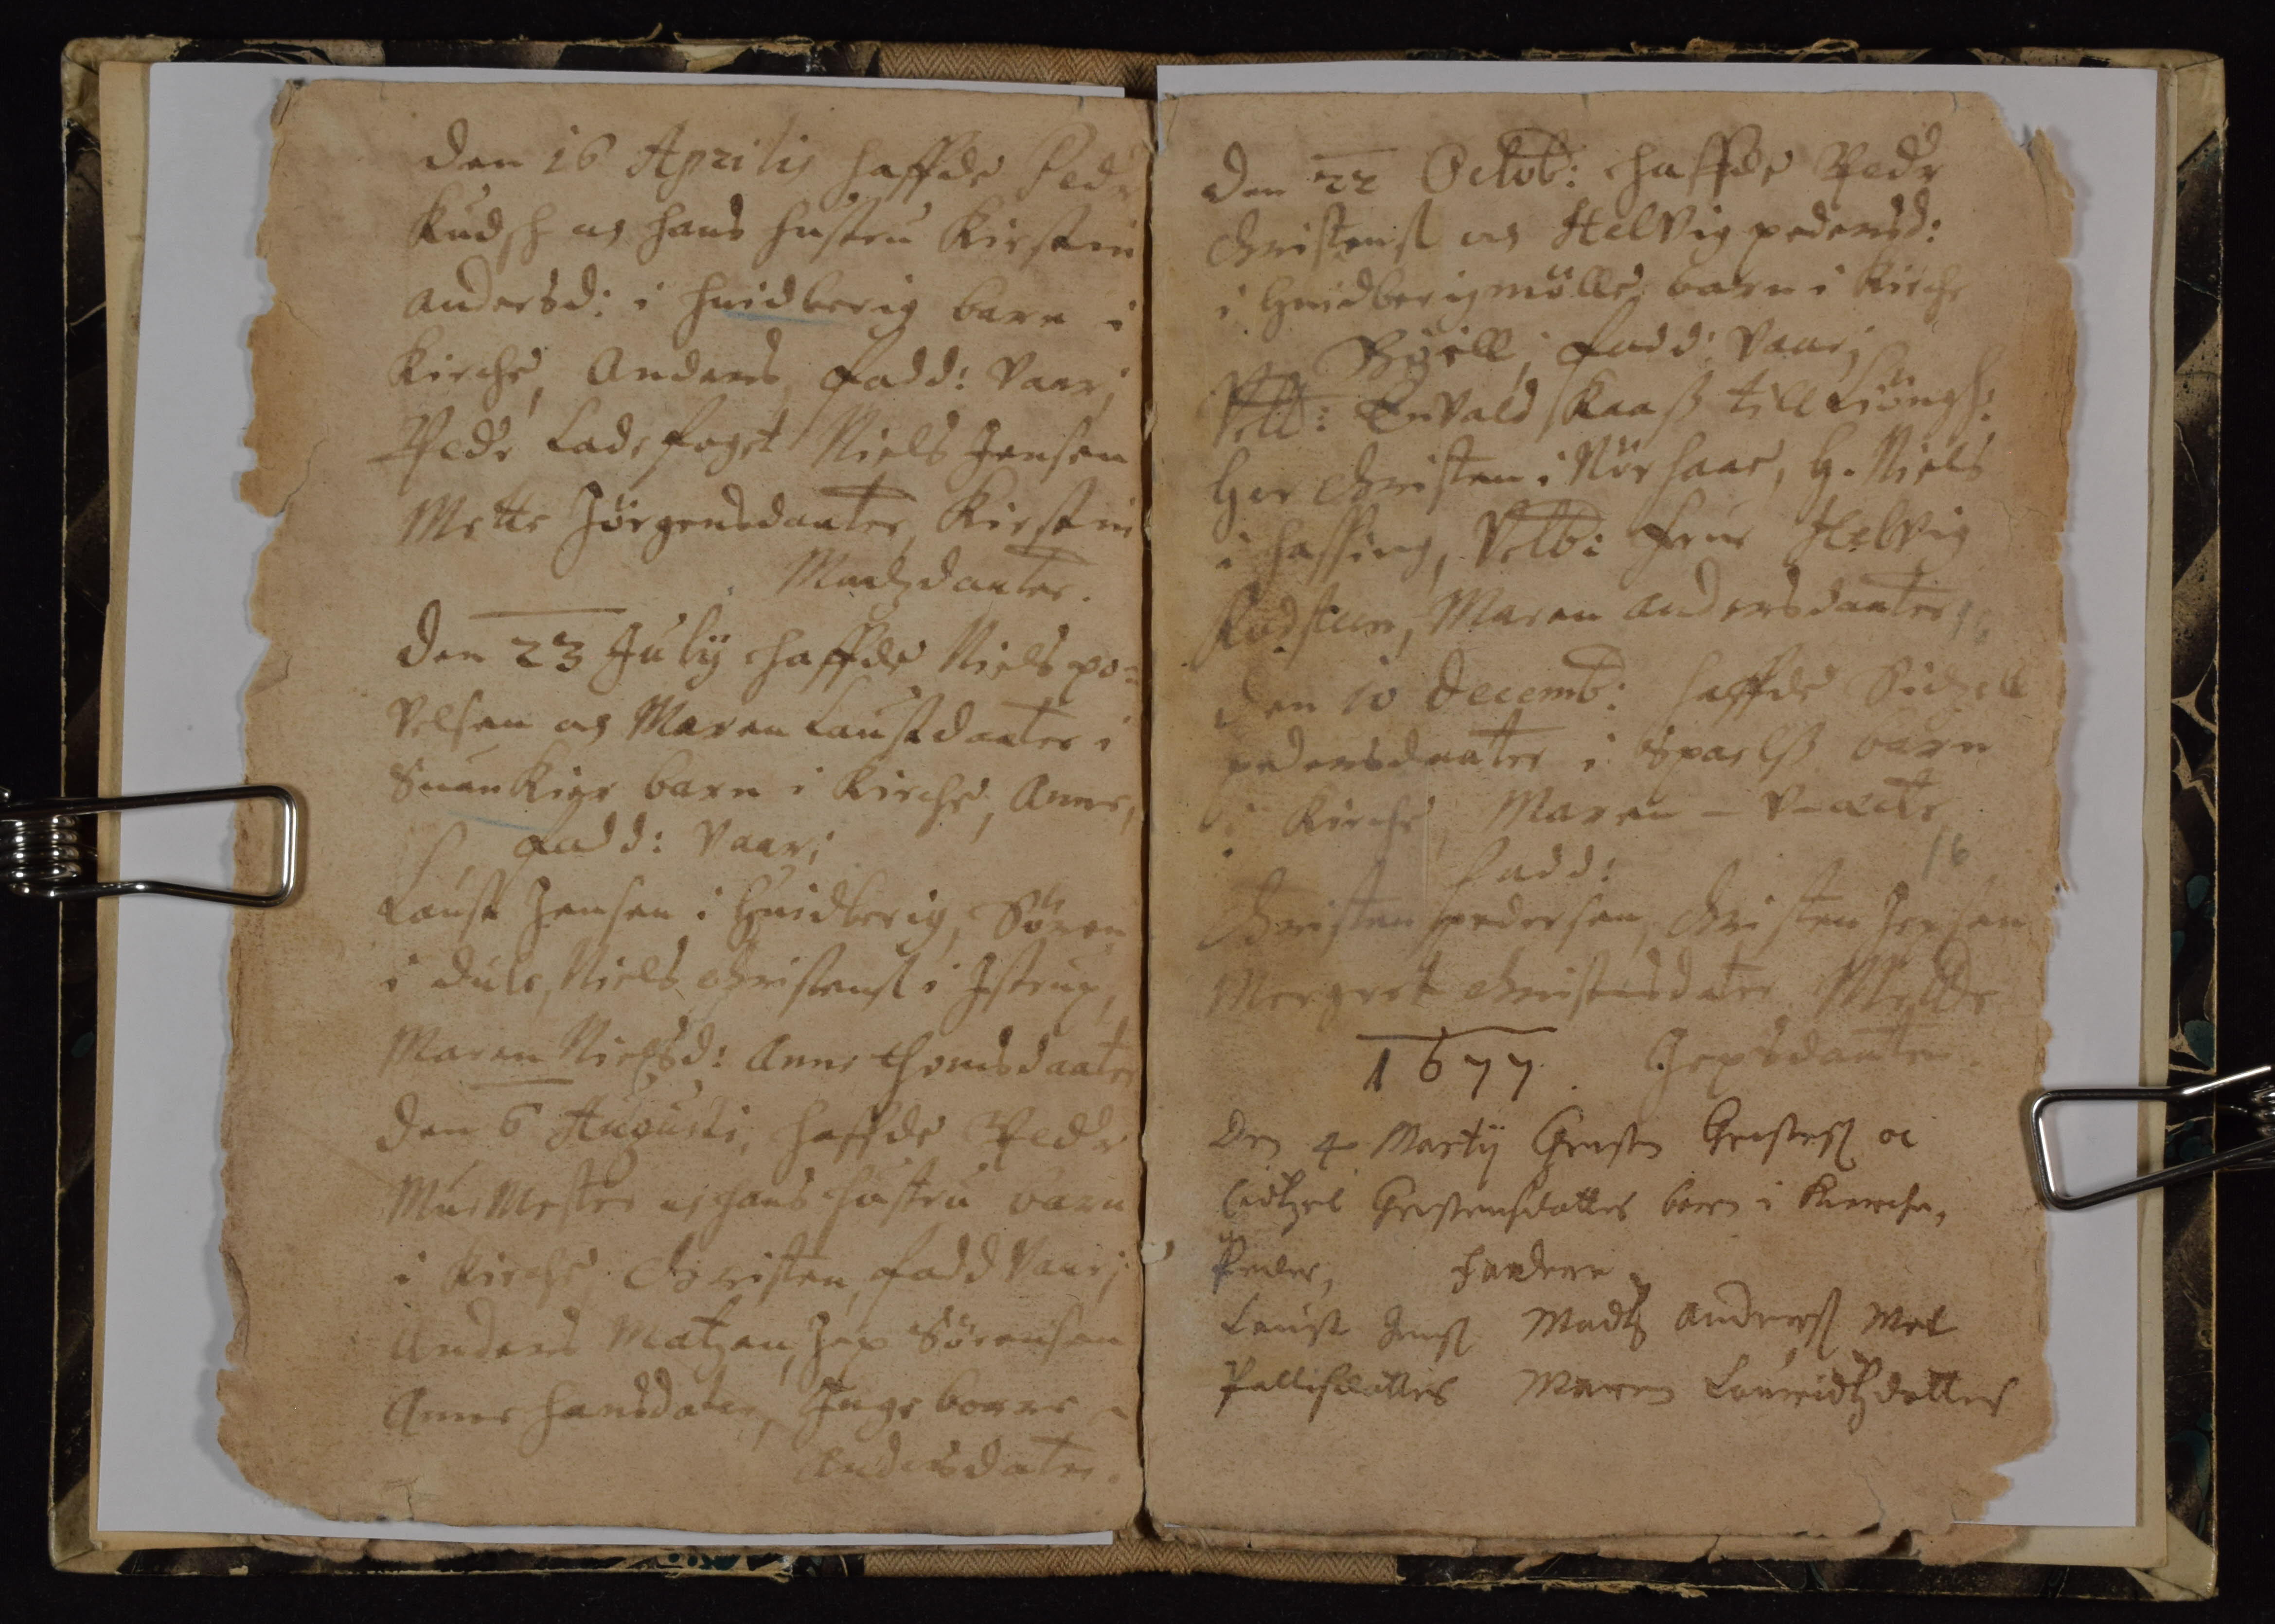Den 16 Aprilis haffde Pedr
kudsh och hans hustru Kirstin
Andersd: i hvidberig barn i
Kirche, Anders, Fadd: vaar
Pedr Ladefoget Niels Jensen
Mette Jørgensdauter Kirstin
Madzdauter.
Den 23 Julij haffde Niels po¬
velsen och Maren Laustdaater i
Suankier barn i Kirche, Anne,
Fadd: vaar;
Laust Jensen i Hvidberig, Søren
i Dule Niels christens i Istrup.
Maren Nielsd: Anne Thomsdaater
Den 6 Augusti, haffde Pedr
Mur Mester och hans hustru barn
i Kirche christen, Fadd vaar;
Anderis Matzen Jep Sørensen
Anne Hansdater Ingeborre
Andersdater
Den 22 Octob: Haffde Pedr
Christens och Helvig Pedersd:
i Hvidberig mølle barn i Kirche
Boell Fadd: vaar
Velb: Envold Kaass till Liøngh:
Her Christen i Nørhaae, H. Niels
i Hassing, Velb: Frue Helvig
Radsteen, Maren Andersdaater
Den 10 Decemb: haffde Sidzell
Pedersdaater i Spaelss barn
i Kirche, Maren - v-eccte
Fadd:
christen Pedersen, christens Jepsen
Mergret christesdater Mette
Jepsdauter
1677
Den 4 Martij Cersten Christensn oc
Cidtzel Christensdatter barn i Kierche,
Peder Faddere
Laust Jens Madtz Anders, Met
Pallisdatter Maren Lauridtzdatter
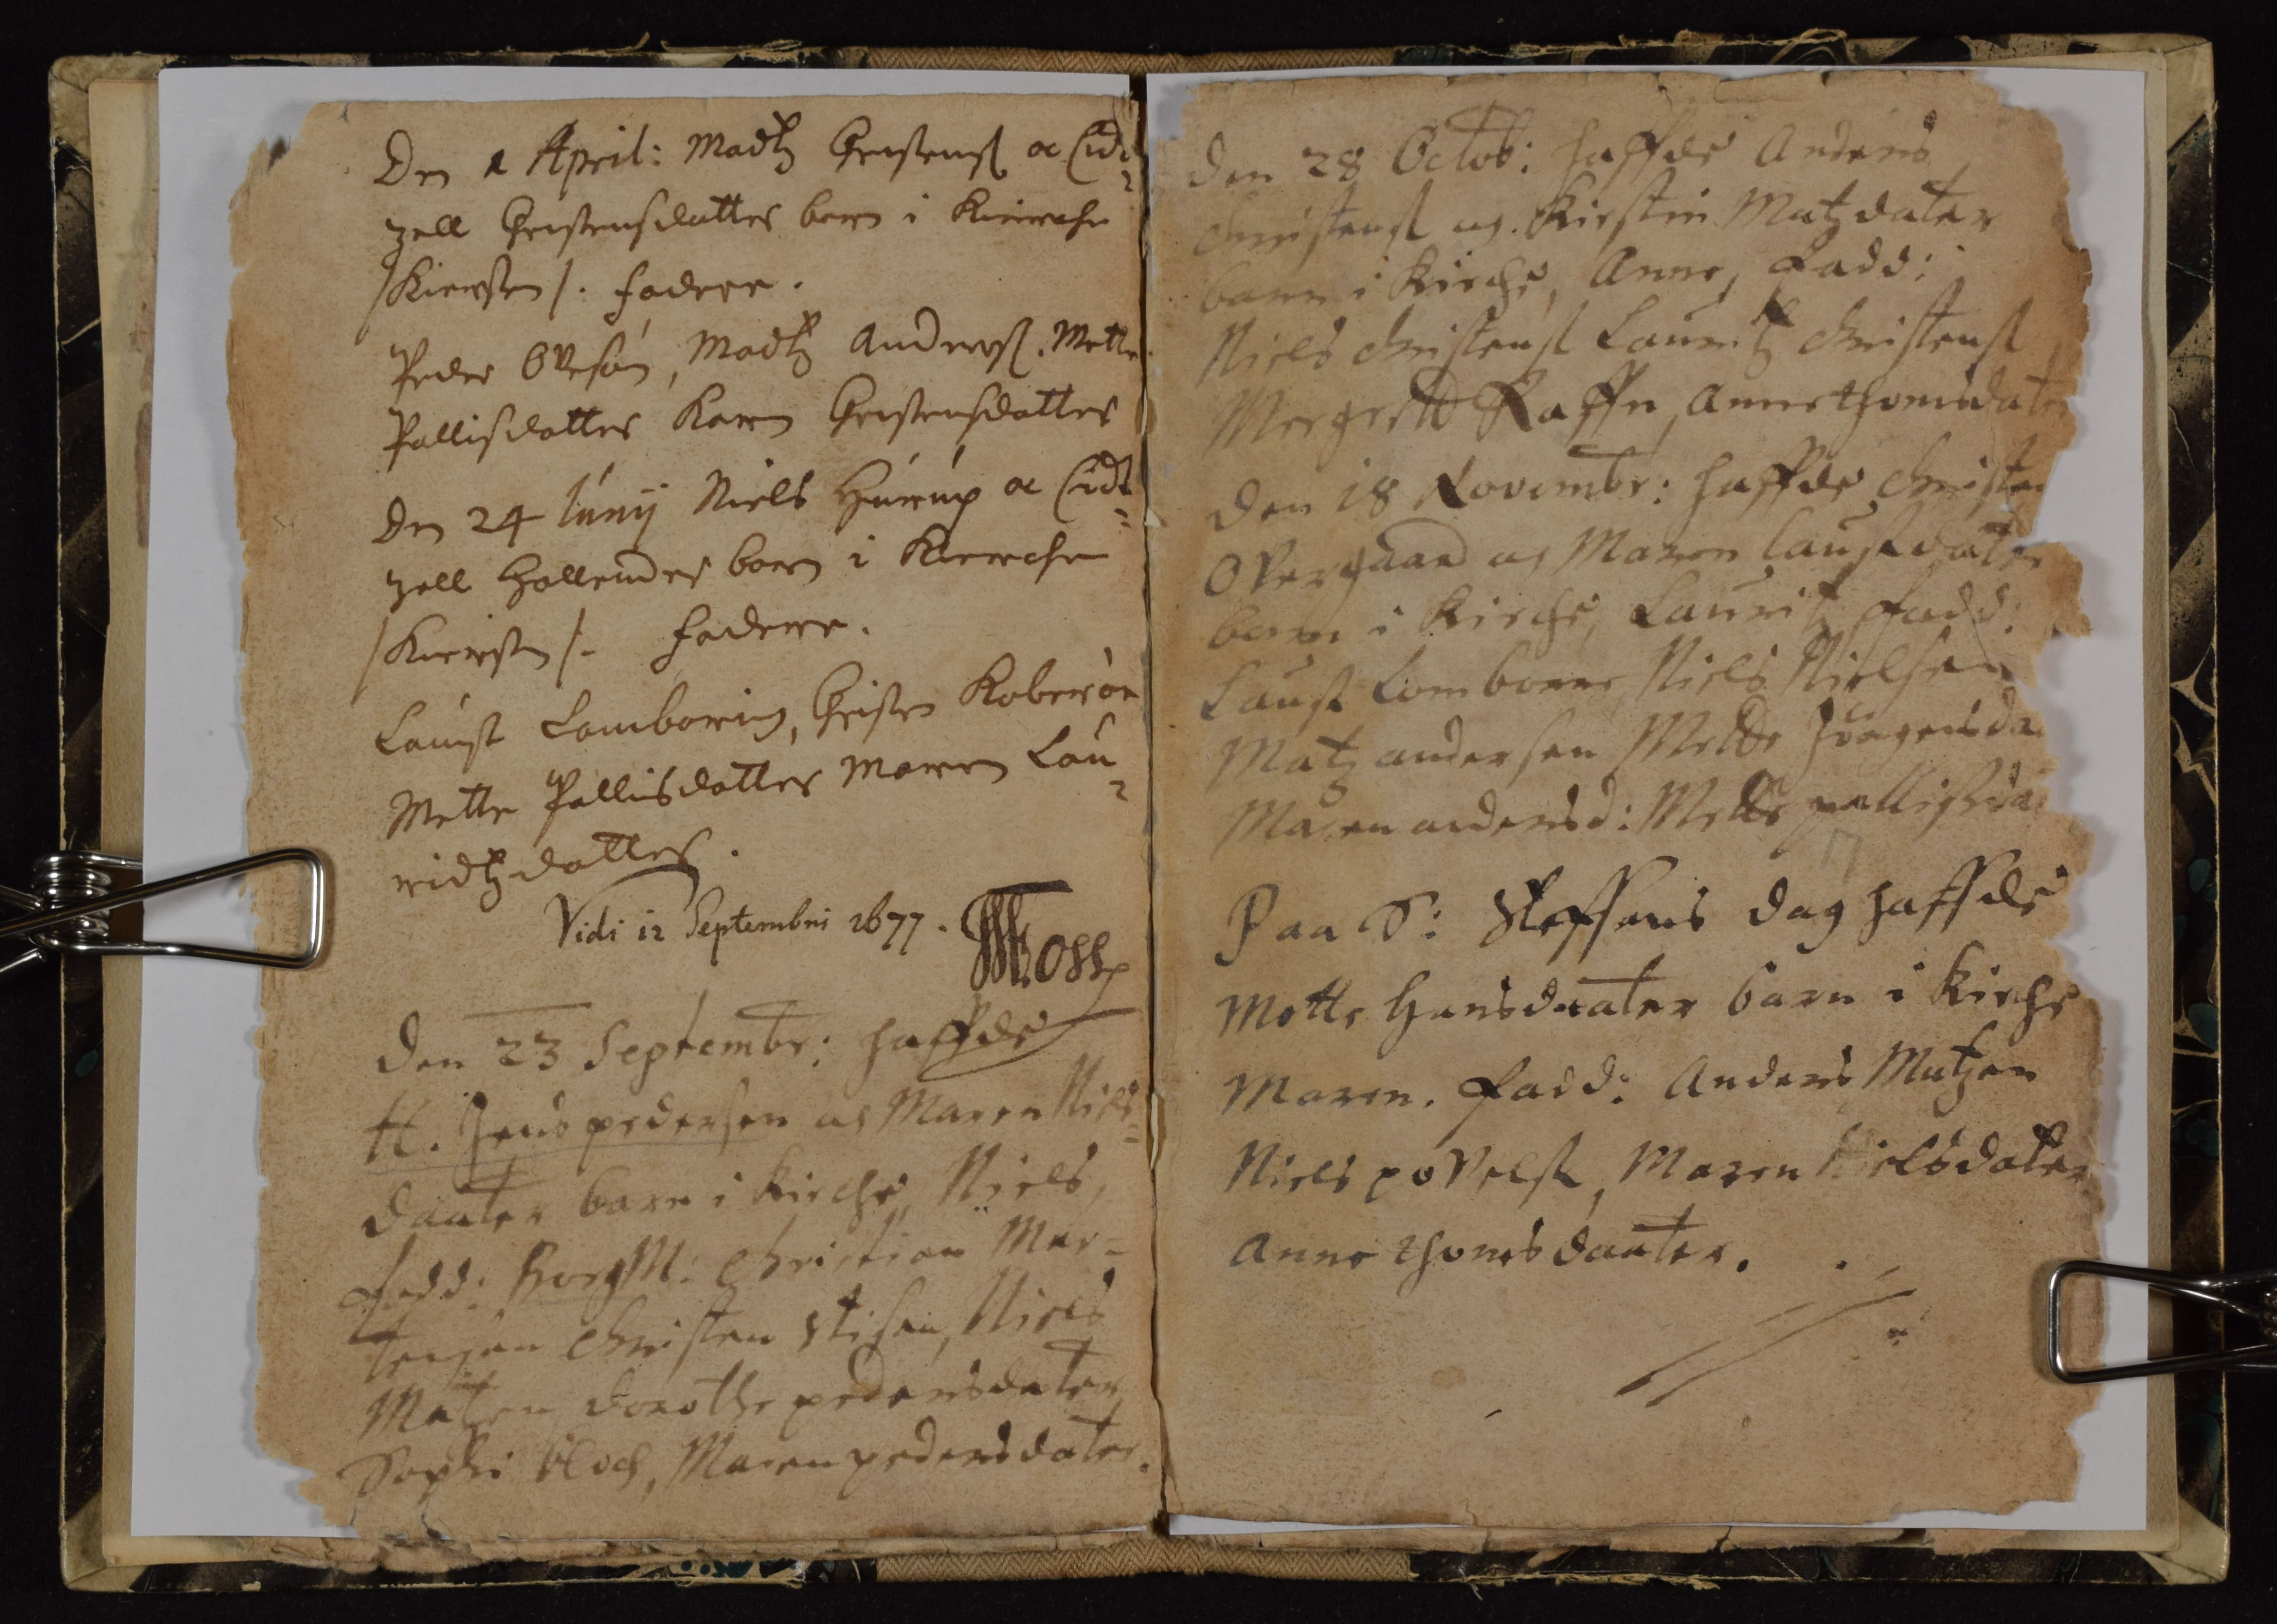Den 1 April: Madtz Christens oc Cid¬
zell Christensdatter barn i Kierche
/ Kiersten /. Fadere
Peder Ovlesøn, Madtz Anders Mette
Pallisdatter Karen Christensdatter
Den 24 Junij Niels Hurup oc Cidt
zell Hollændes boern i Kirche¬
/ Kiersten /. Fadere.
Laust Lomborig Christen Roberøn
Mette Pallisdatter Marren Lau¬
ridtzdatter.
Vidi 12 Septembri 1677
##oss
Den 23 Septembr: haffde
H. Jens Pedersen och Maren Niels¬
dauter barn i Kirche, Niels,
Fadd: RoegM. Christan Mar¬
tensen Christen Stisen, Niels
Matzen Dorothe pedersdater
Sophi bloch, Maren pedersdater
Den 28 Octob: haffde Anders
Cristens och Kirstin Matzdater
barn i Kirche, Anne, Fadd:
Niels christens Lauritz christens
Mergete Raffn Anne thomsdater
Den 18 Novembr: haffde Christen
Overgaard och Maren Lausdater
barn i Kirche, Lauritz, Fadd:
Laust Lomborre Niels Nielsen
Matz andersen Mette Jørgensda
Maren Andersd: Mette pallisda
Paa S: Steffans dag haffde
Mette Hansdaater barn i Kiche
Maren. Fadd: Anders Matzen
Niels povels, Maren Nielsdater
Anne thomes daater